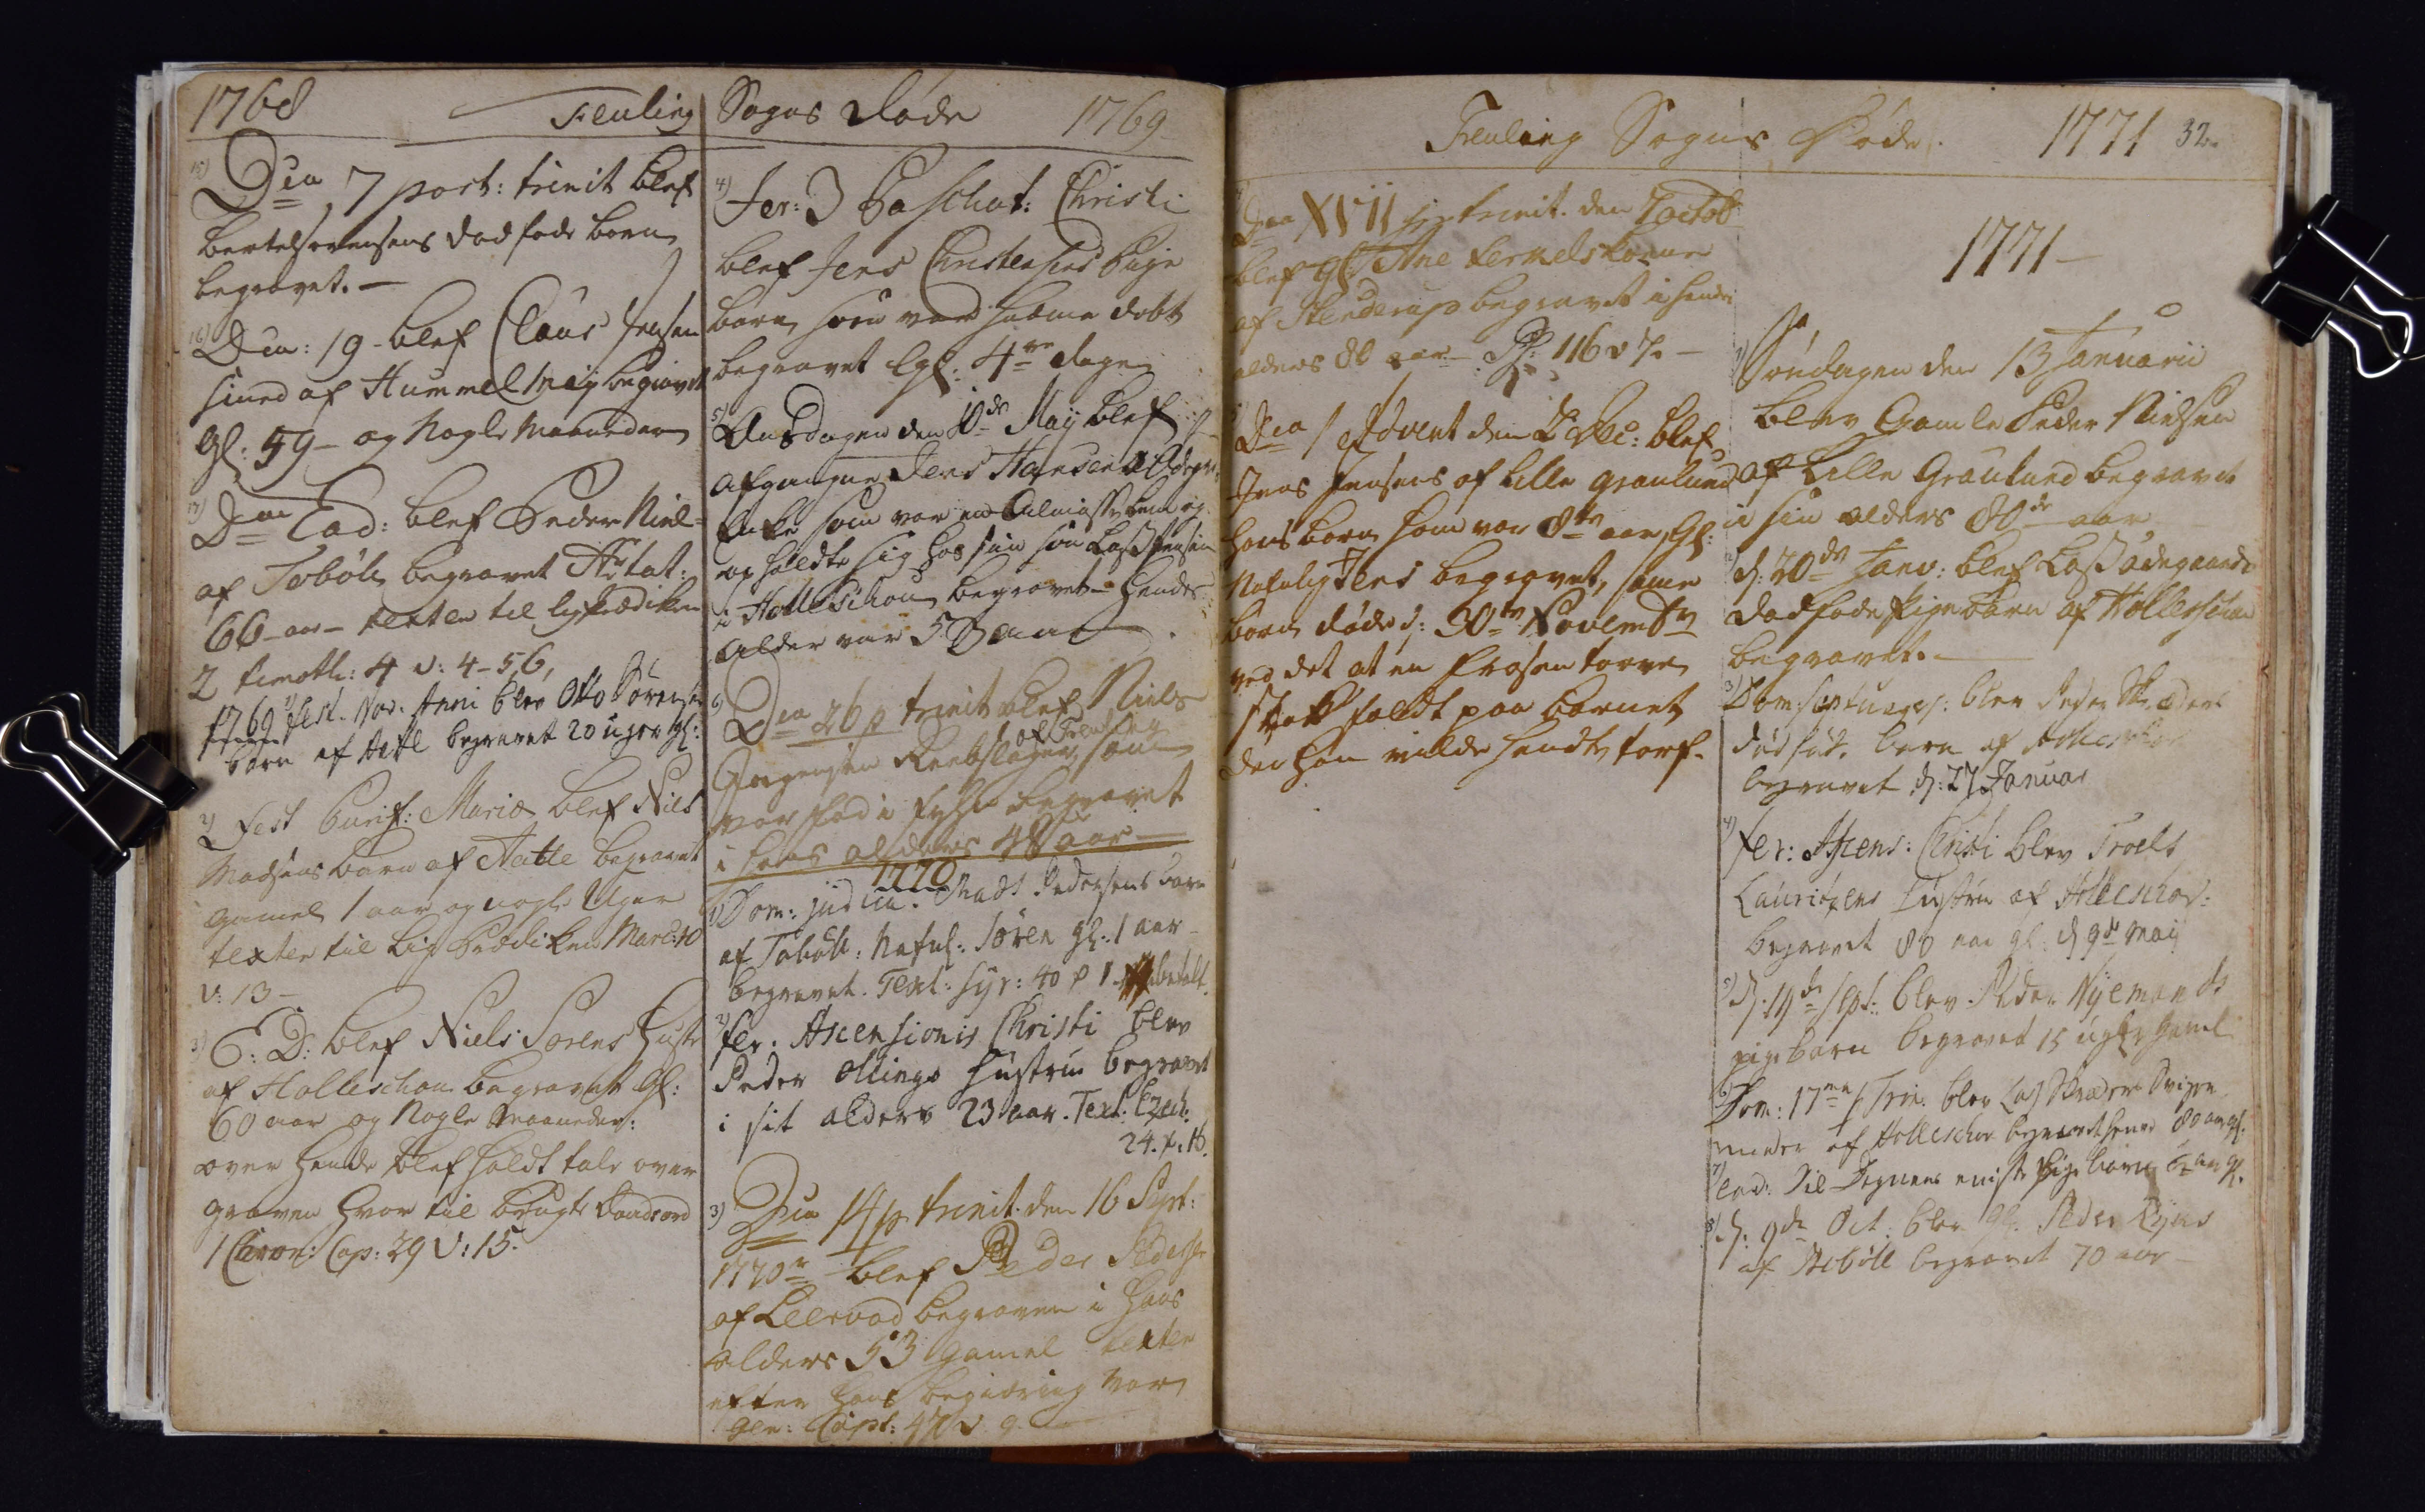1768
Feuling Sogns Døde
15)
Dca 7 post: Trinit blef
Bertel sorensens dodfode barn
begravet. -
16)
Dca: 19. blef Claus Jensen
sined af Hummel## begravet
gl: 59 -  og nogle Maaneder
17)
Dca Eod: blef Peder Niel
af Tobøll begravet Ar tat:
66 - aar - texten til Liig Prædiken
2 fimotl: 4 v: 4-5,6
1)
1769 Fest: Nov: Anni blev Otto Sørensens
Barn af Aatte begravet 20 uger gl:
2) Fest Purif: Mariæ blef Niels
Madsens Barn af Aatte begravet
Gammel 1 Aar og nogle Uger
texten til Lig prædiken Marc: 10
v: 13
3) E: D: Blef Niels Sørens Hustr
af Holleschou begravet gl:
60 Aar og nogle Maaneder:
over Hende Blef holdt tale over
graven hvor til brugte Davids: ord
1 Chroe: Cap: 29 v: 15.
1769.
4)
Fer: 3 Paschat: Christi
blef Jens Christensens Pige
barn som var hiæme dobt
begravet Gl: 4re dage.
5)
Onsdagen den 10de Maij blef
afgangne Jens Hansen ##
Enke som var en Almisse Lem og
op holdte sig hos sin søn Lass Jensen
i Holleschou begravet hendes
Alder var 53 Aar.
6)
Dca 26 p. Trinit blef Niels
af Feuling
Jorgensen Reebslager, som
var fod i Fyhn begravet
i hans alders 48 aar
1770
1) Dom: Judica. Madt Pedersens barn
af Tobøll: Nafnl: Søren gl: 1 Aar
begravet Text: Syr: 40 p. 1 ## betalt
2)
Fer. Ascensionis Christi blev
Peder Ollings Hustrue begravet
i sit alders 23 Aar. Text. Ezech:
24. f: 16
3) Dca 14 p Trinit. den 16 Sept:
1770## blef Peder Pedersen
af Leervad begraven i Hans
alders 53 Gamel Texten
efter Hans begiæring vare
Gle Capt: 47 v 9
Feuling Sogns Døde
4)
Dca XVII ## Trinit. den 7 octob
blef gl: Ane Terkels Koene
af Stenderup begravet i hendes
Alders 8 Aar. PL: 116 v 7##
5)
Dca 1 Advent den 2 Dec: blef
Jens Jensens af Lille Graulund
hans barn som var 8te en gl:
Nanafnlig Jens begravet, same
barn døde d: 30te Novembr
ved det at en frosen torve
stak faldt paa barnet
da han vilde handte torf.
32.
1771
1771
1)
Søndagen den 13 Januarii
blev Gamle Peder Nielsen
af Lille Graulund begravet
af sin alders 80de aar.
2)
d: 20de Janv: bleff Lass ødegaards
dodfode Pigebarn af Holleschou
begravet.
3)
Dom: septuages: blev Peder Skræders
dødfød barn af Holleschov
begravet d: 27 Januar
4)
Fer: Ascens: Christi blev Troelss
Lauritzens Hustrue af Holleschov:
begaaet 80 Aar gl: d 9de Maij
5)
d: 19de Sept: blev Peder Nyemands
pigebarn begravet 15 uger gaml.
6)
Dom: 17ma p Trin: blev Las Skræders Sviger
moder af Holleschov begravet henved 80 aar gl.
7)
Eod: Die Degnens eniste Pigebarn ## gl:
8)
d: 9de Oct. blev gl: Peder Ryks
af Bobøll begravet 70 aar
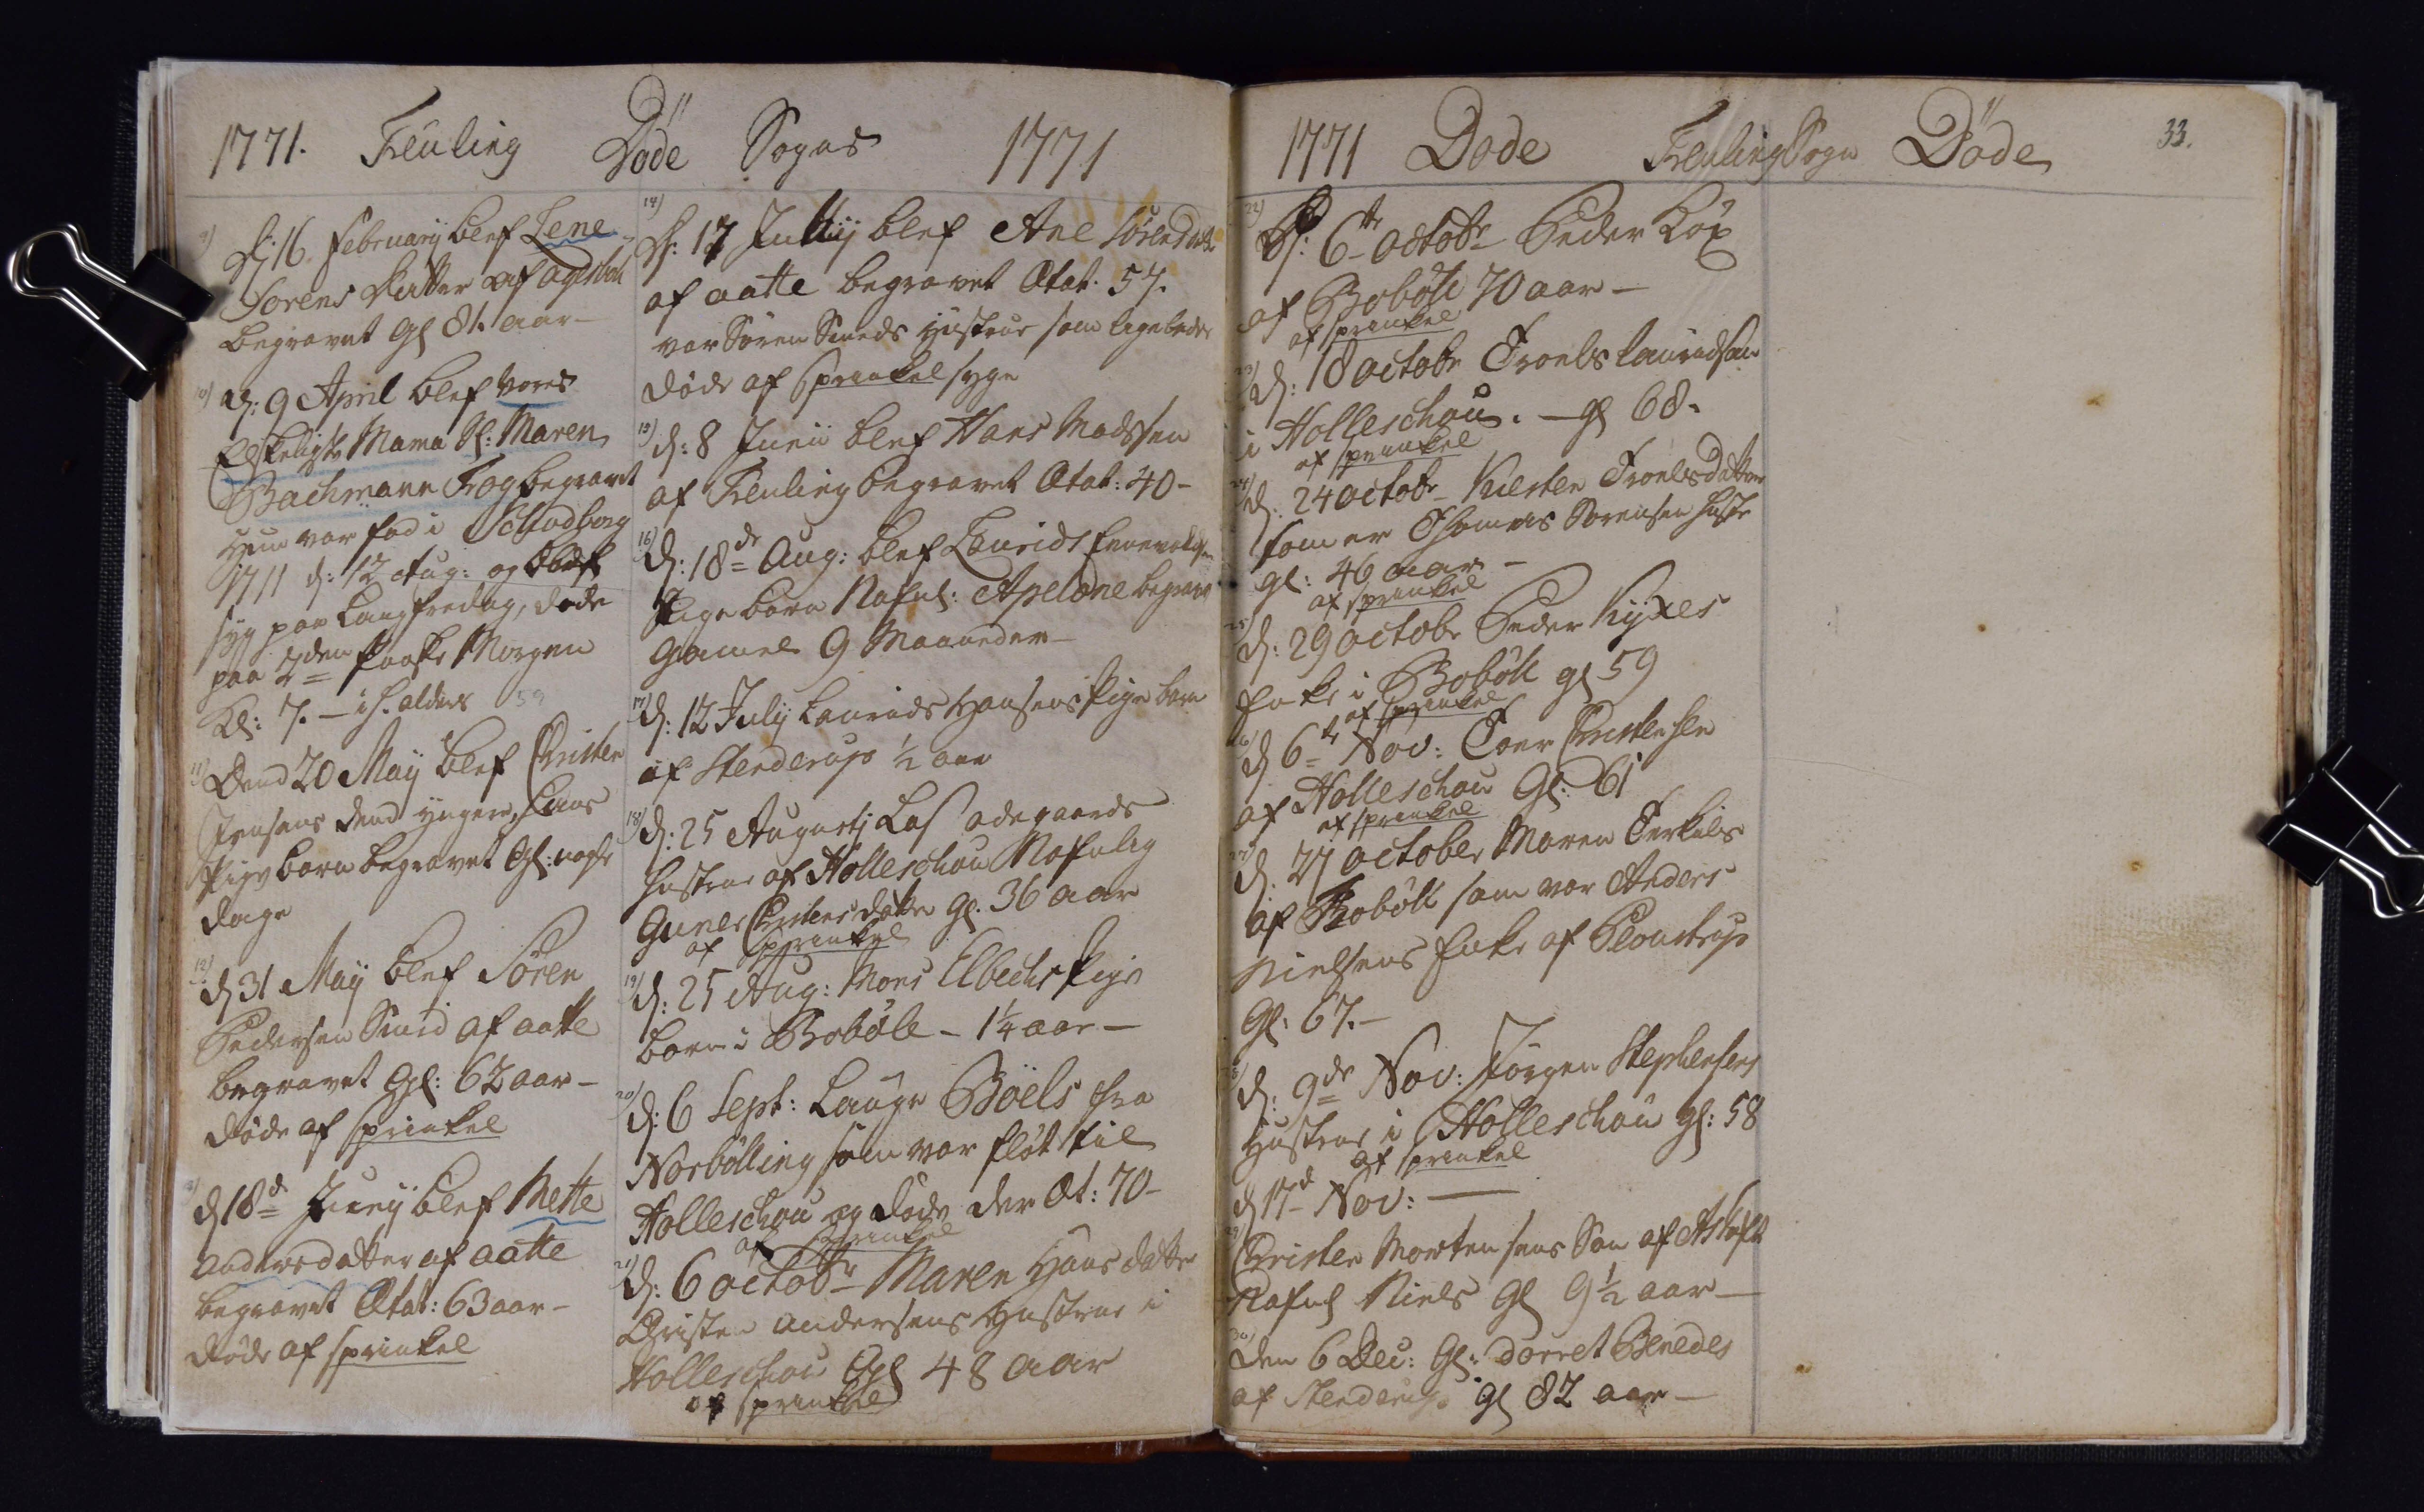1771. Feuling Døde Sogns
9)
D: 16. Februar: blef Lene
Sorens Datter af Agesboll
begravet Gl 81. Aar
10) d: 9 April blef vores
Eskeligste Mama Sl: Maren
Bachmann Fog begravet
Hun var fod i Schodborg
1711 d: 12 Aug: og blef
syg paa Langfredag dode
paa 2den Paaske Morgen
Kl: 7. i h. alders 59
11) Dend 20 Maij blef Christen
Jensens dend yngere, hans
Pigebarn begraven Gl: nogle
Dage
12)
d 31 Maij blef Søren
Pedersen Smid af aatte
begravet Gl: 62 aar -
døde af sprinkel
13)
d 18de Junij blef Mette
Andersdatter af Aatte
begravet Ætat: 63 Aar
døde af Sprinkel
1771
14)
D: 14 Jullij blef Ane Sørensdatter
af aatte begravet Ætat: 57.
var Søren Smeds Hustrue som ligeleded
døde af sprinkel syge
15)
d: 8 Junii blev Hans Madsen
af Feuling begravet Ætat: 40 -
16)
d: 18de Aug: bleff Laurids Eenevoldsens
Pigebarn Nafnl: Apelone begravet
Gammel 9 Maaneder -
17)
d: 12 Julij Laurids Hansens Pige barn
af Stenderup ½ aar
18)
d 25 Augusti Las odegaards
Hustrue af Holleschou Nafnlig
Guner Christensdatter gl: 36 Aar
af sprinkel
19)
d: 25 Aug: Mons Elbechs Pige
Barn i Bobøll - 1¼ aar -
20)
d: 6 Sept: Lauge Boels fra
Norbølling som var fløt til
Holleschou og døde der Æt: 70 -
af sprinkel
21)
d: 6 Octobr Maren Hansdatter
Christen Andersens Hustrue i
Holleschou Gl 48 aar
af sprinkel
1771 Dode Feuling Sogn Døde
22)
d: 6 Octobr Peder Kop
af Bobøll 70 Aar -
af sprinkel
23)
d: 10 Octobr Troels Lauridsen
i Holleschou. gl 68.
af sprinkel
24)
d: 24 Octobr Kiersten Troelsdatter
som var Thomas Sørensen Hustr
gl: 46 aar
af sprinkel
25)
d: 29 Octobr Peder Ky##es
Enke i Bobøll gl 59
af sprinkel
26)
d 6te Nov: Coer Christensen
af Holleschou gl: 61
af sprinkel
27)
d: 27 October: Maren Terkels¬
af Bobøll som var Anders
Nielsens Enke af Ploustrup
gl: 67. -
28)
d: 9de Nov: Jørgen Stephensens
Hustrue i Holleschou gl: 58
af sprinkel
d 17de Nov:
29)
Christen Mortensens Søn af Astoft
Nafnl Niels gl 9½ aar -
30)
den 6 Dec: Gl: Dorret Benedes
af Stenderup gl 82 Aar -